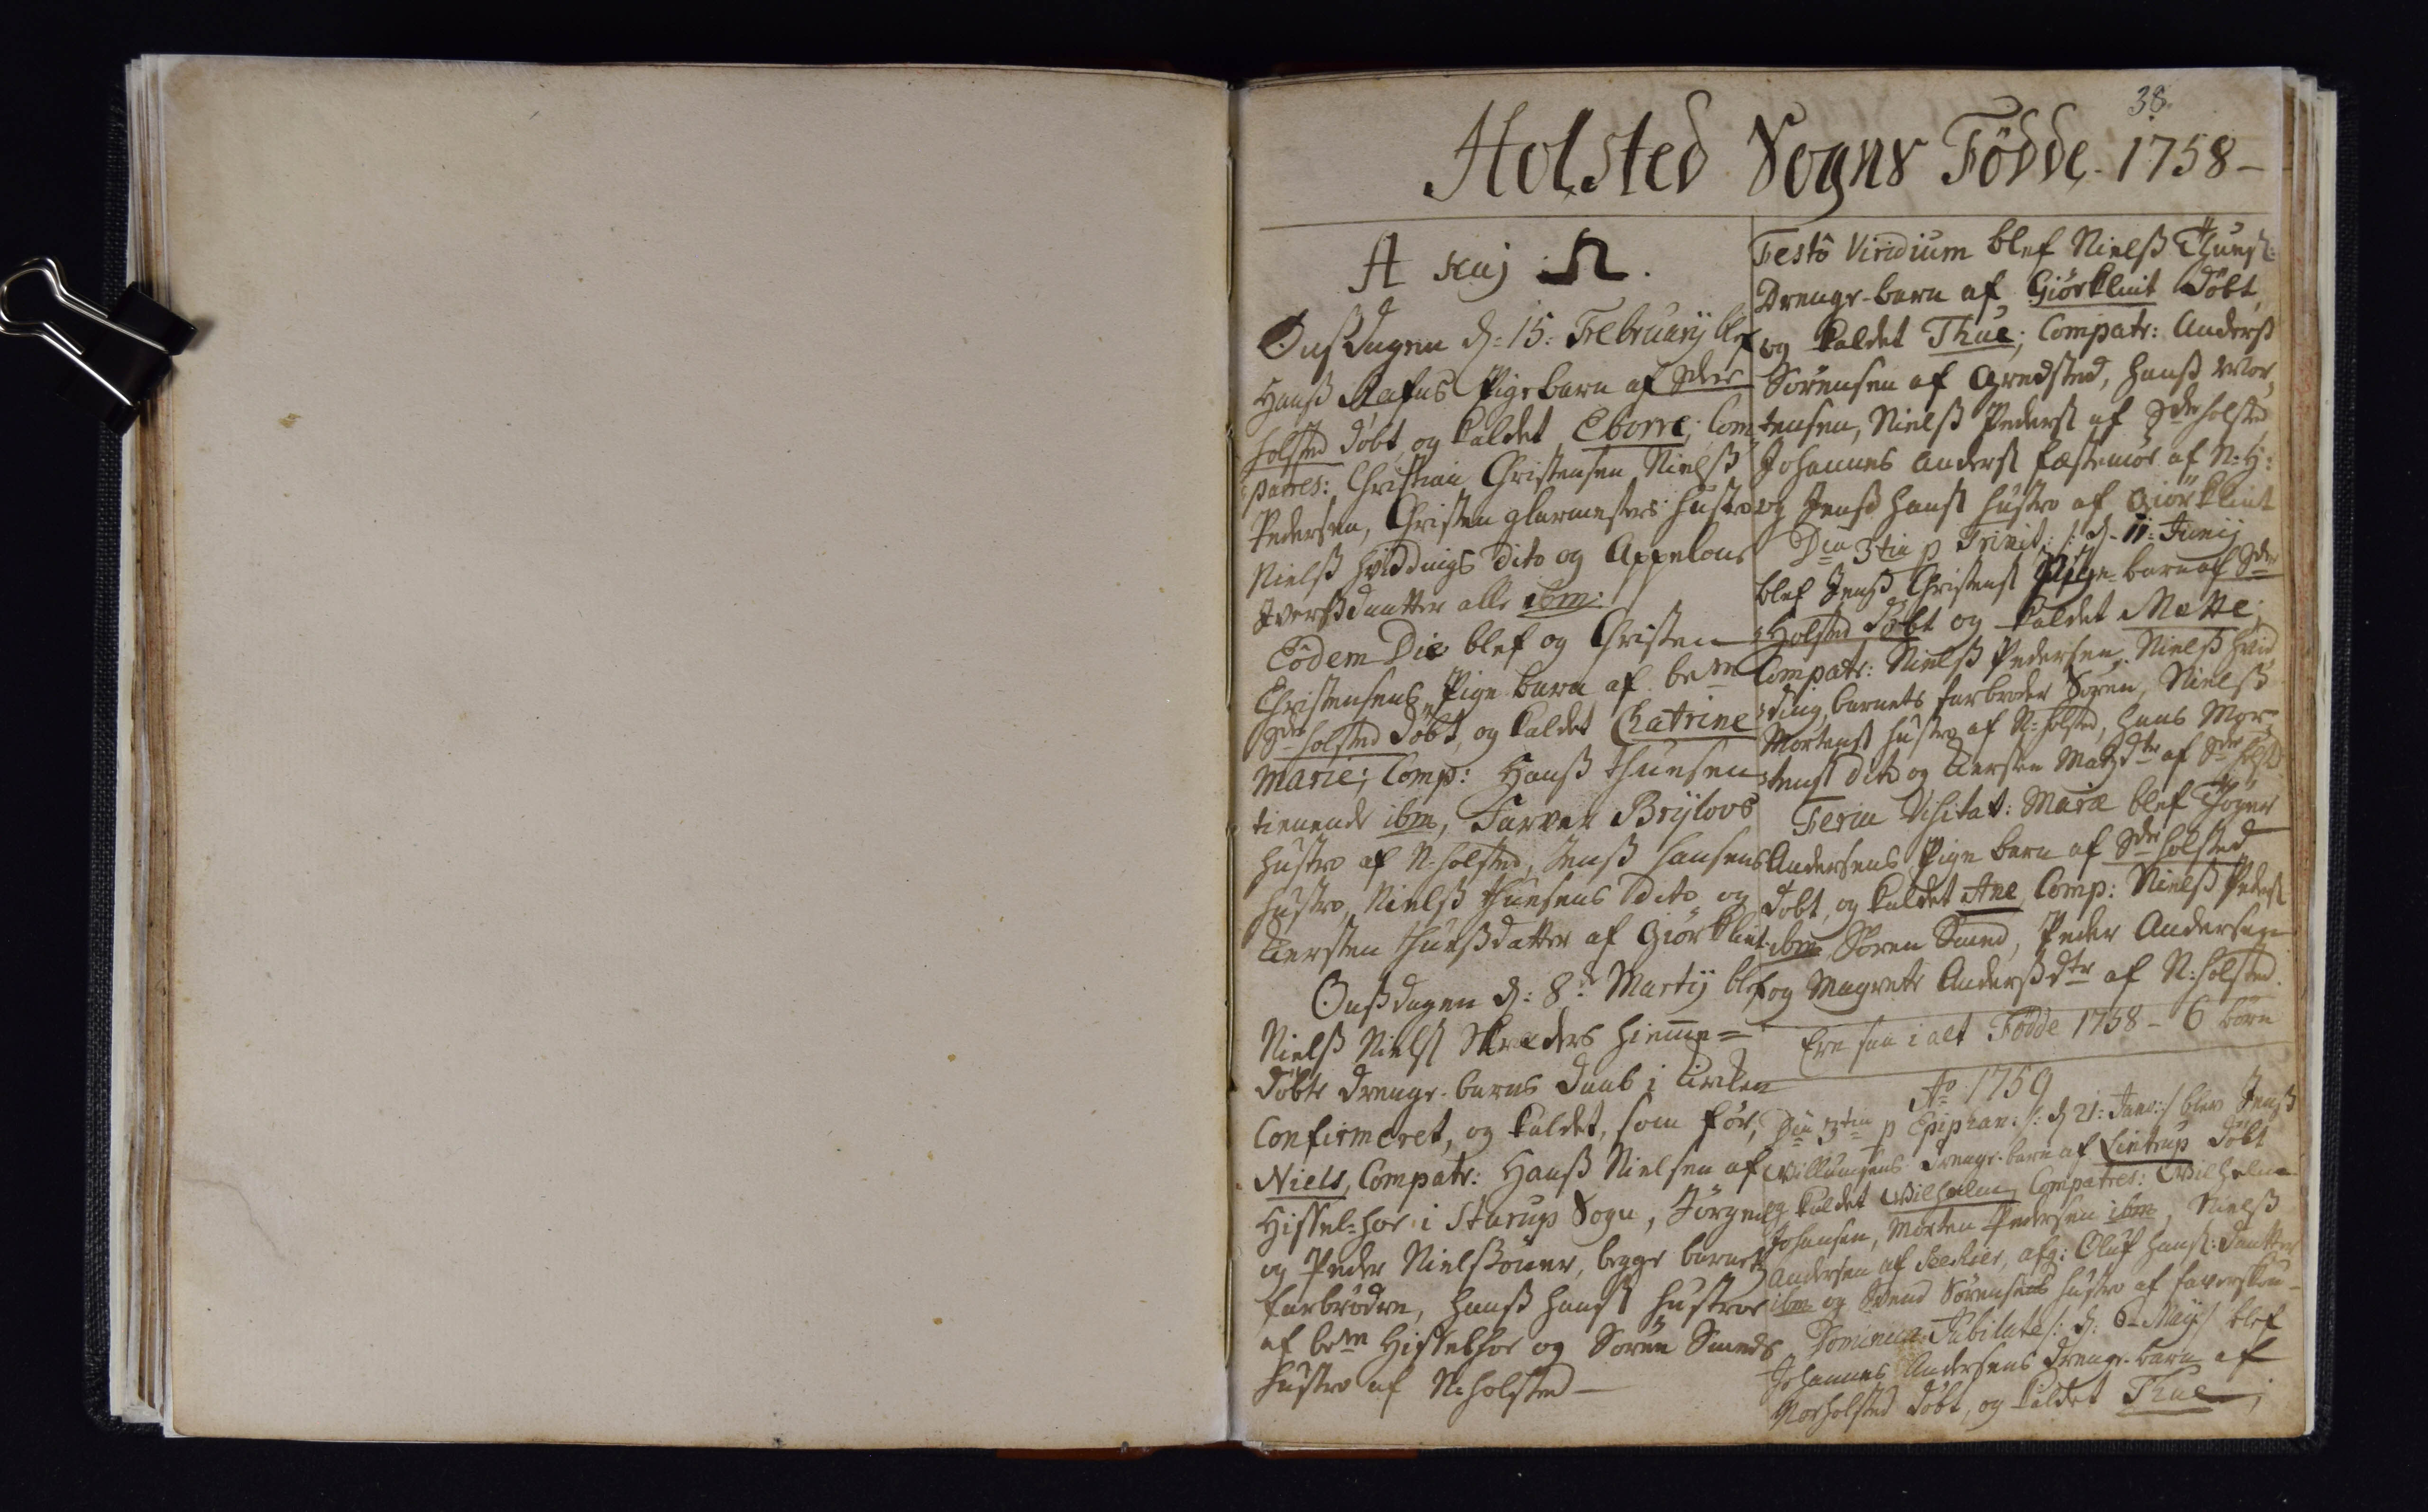Holsted Sogns Fødde. 1758 -
A Kaj Ω
Onssdagen d: 15: Februarij blef
Hans Rafns Pigebarn af Sdr
holsted døbt og kaldet Eborre; Com¬
parres: Christian Christensen Nielss
Pedersen, Christen glarmesters Hustro
Niels hviddings dito og Appelone
Iverfsdaatter alle ibm:
Eodem Die blef og Christen
Christensens Pige barn af bem
Sdr
holsted døbt og kaldet Chatrine
Marie; Comp: Hanss thuesens
tienende ibm: Farver Brylovs
Hustro af N: holsted, Jenss Hansens
Hustro Nielss Thuesens dito og
Kiersten Tuessdatter af Giørklint
Onsdagen d: 8de Martij blev
Nielss Nielss Skræders hiemme¬
døbte Drenge-barns daab i Kirken
Confirmeret, og kaldet, som før,
Niels Compatr: Hanss Nielsen af
Hissel=hoe i Starup Sogn; Jørgen
og Peder Nielsøner, begge barnets
farbrødre, Hanss Hanss Hustroe
af bem Hisselhoe og Søren Smeds
hustru af N: holsted -
38.
Festo Viridium blef Nielss Thues:
Drenge-barn af Giørklint døbt,
og kaldet Thue, Compatr: Anderss
Sørensen af Gredsted, Hans Mor¬
tensen, Niels Peders af Sdr holsted
Johannes Anders fæstemøe af N: H:
og Jenss Hans Hustro af Giørklint
Dca 3tia p Trinit: /: d. 11: Junij
blef Jenss Christens Pige-barn af Sdr
Holsted døbt og kaldet Mette;
Dompatr: Nielss Pedersen, Nielss Hvid¬
ding, barnets farbroder Soren, Niels
Mortens Hustro af N: holsted, Hans Mor¬
tens dito og Kirsten Madzdtr af Sdr holsted
Feria Visitat: Mariæ blef Thøger
Andersens Pige barn af Sdr holsted
døbt og kaldet Ane Comp: Niels Peders
ibm Søren Smed, Peder Andersen
og Magrete Andersdtr af N: holsted
Ere saa i alt Fødde 1758 - 6. børn
Ao 1759
Dca 3tia p Epiphan: /: d 21 Janv: :/ blev Jenss
Villumsens Drenge-barn af Lintrup døbt
og kaldet Wilhelm, Compatres. Wilhelm
Johansen, Morten Pedersen ibm; Nielss
Andersen af Seekier, afg: Oluf Hanss: Daatter
ibm og Svend Sørensens Hustro af faverskou
Dominica Jubilate /: d: 6 Maij blef
Johannes Andersens Drenge-barn af
Norholsted døbt, og kaldet Thue;
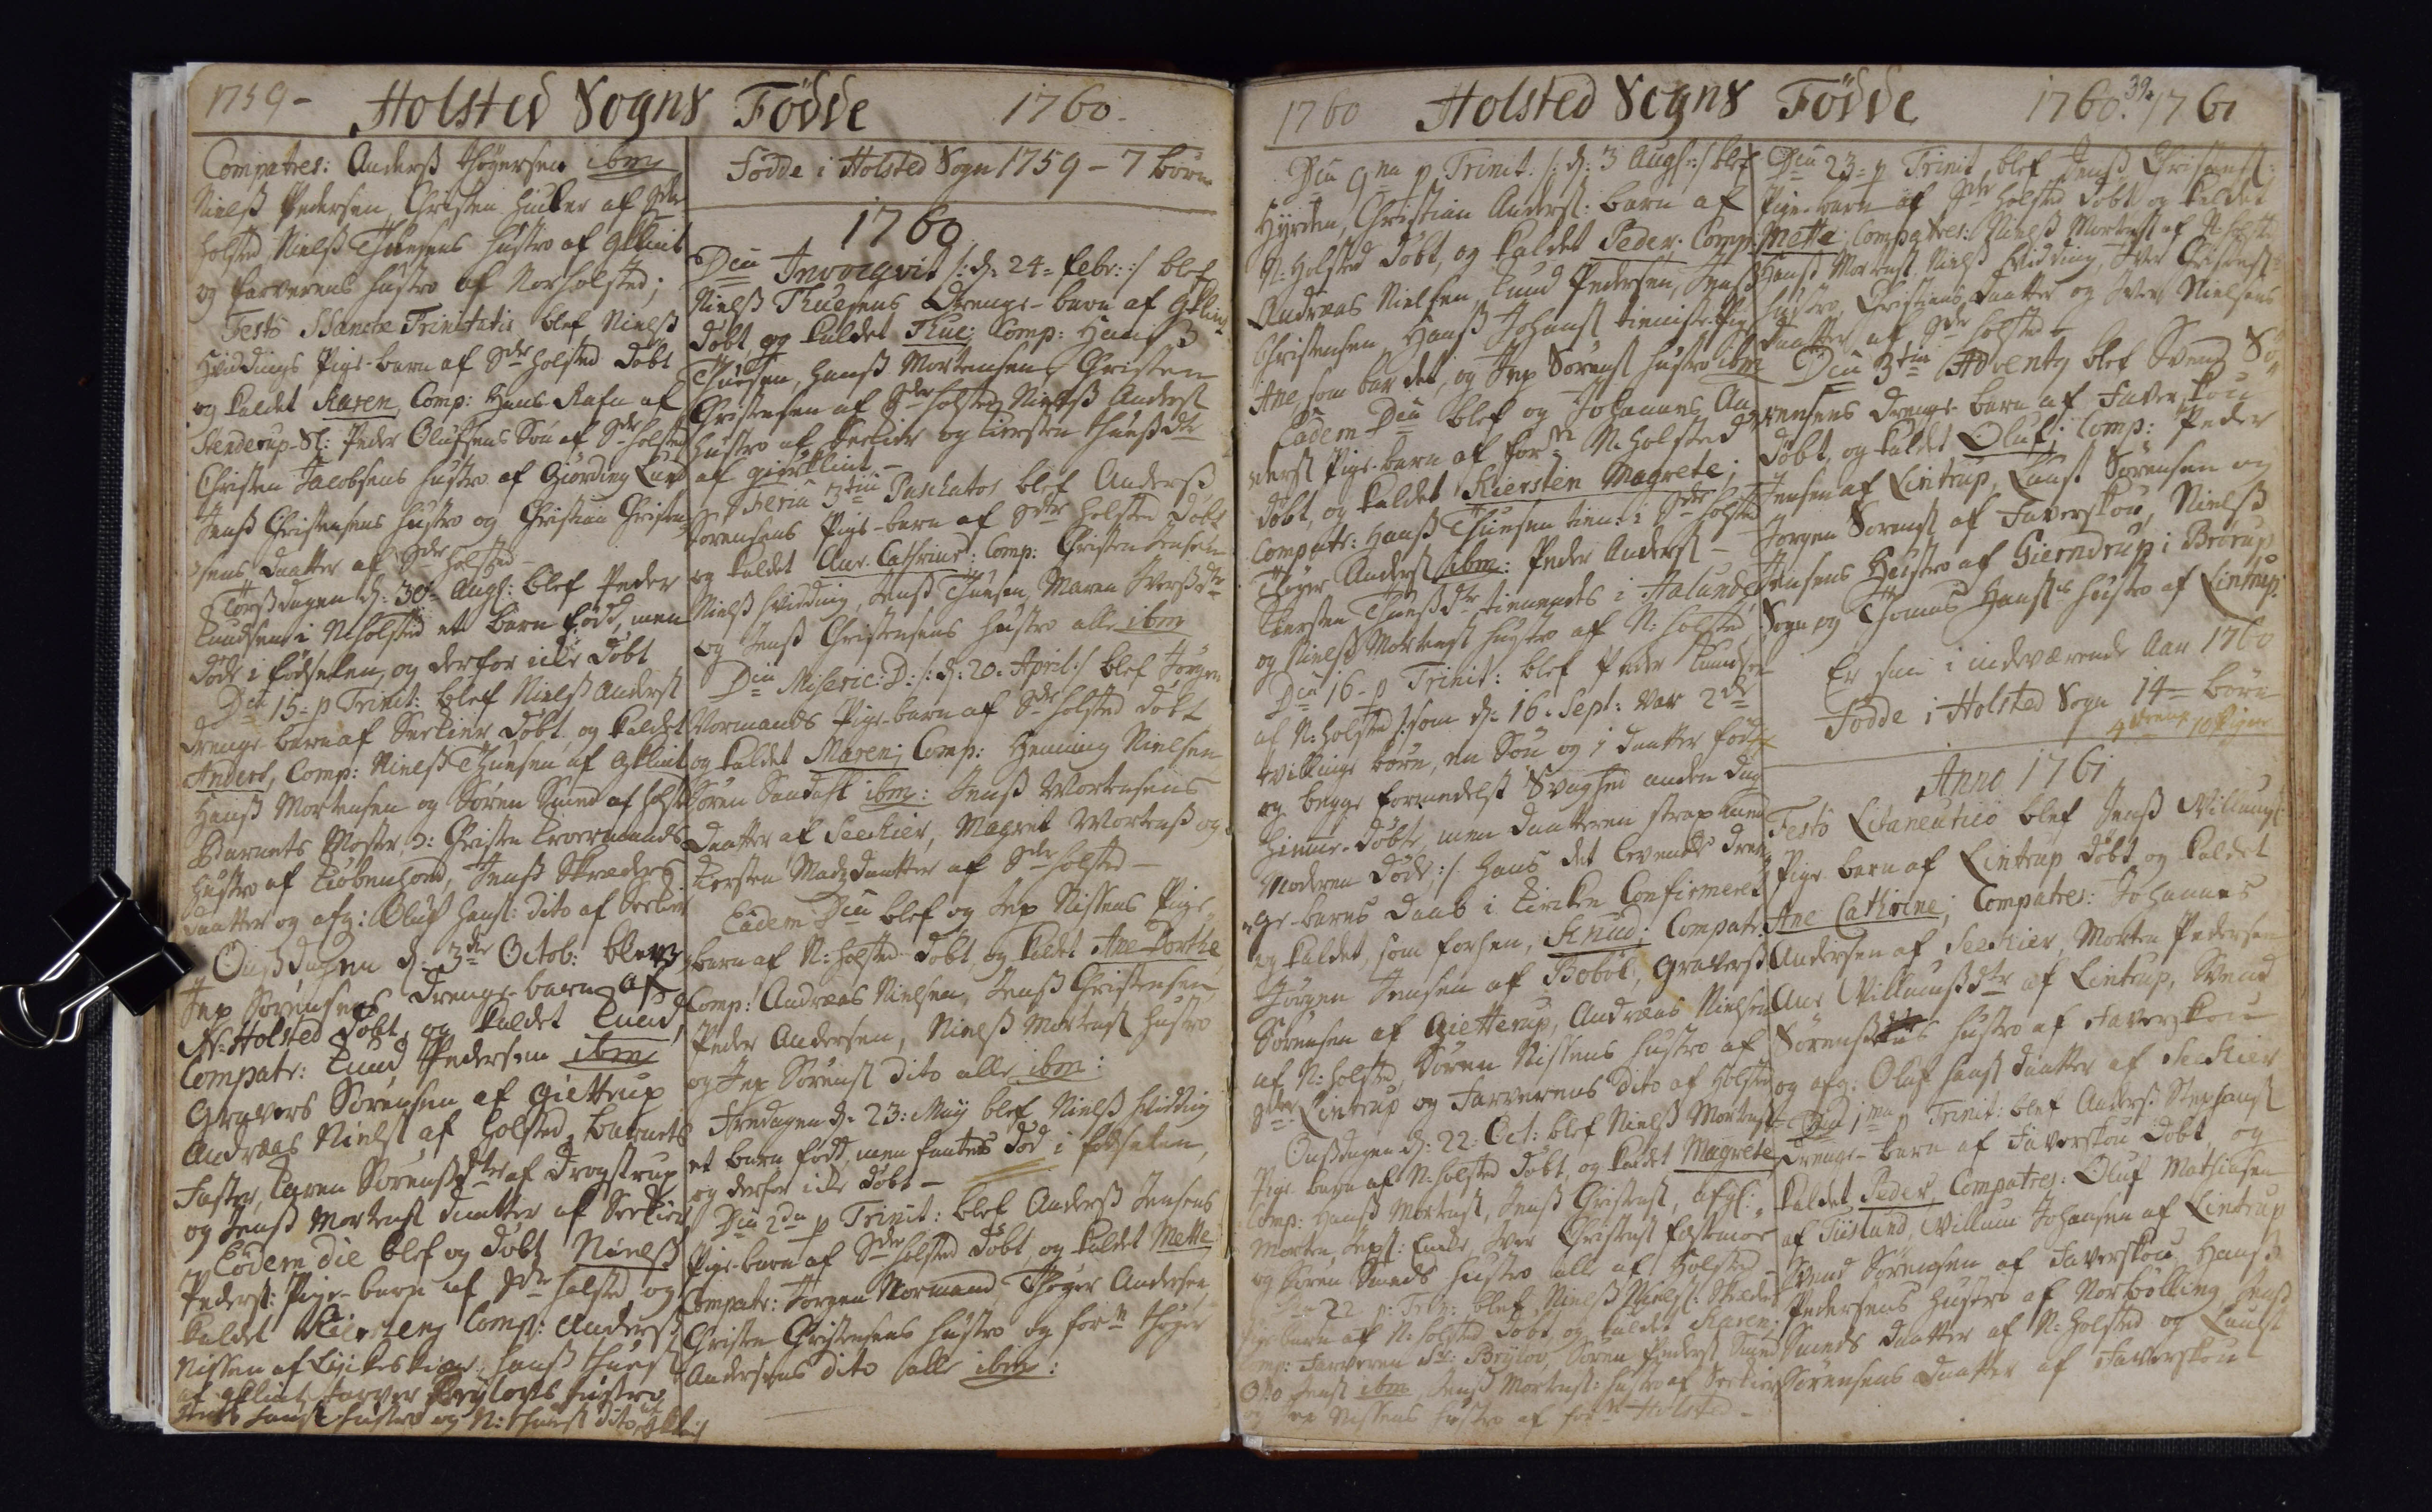1759 -
Holsted Sogns Fødde
Compatres: Anders Thøgersen ibm
Nielss Pedersen Christen Hiuler af Sdr
Holsted Niels Thusens Hustro af Gklint
og farverens Hustro af Nor Holsted
Festo SSancte Trinitatis blef Nielss
Hviddings Pige-barn af Sdr Holsted døbt
og kaldet Karen, Comp: Hans Nafn af
Stenderup - Sl: Peder Olufsens Søn af Sdr Holsted
Christen Jacobsens Hustro af Giørding Lund
Henss Christensens Hustro og Christian Christen¬
sens Daatter af Sdr Holsted -
Torssdagen d: 30: Aug: blef Peder
Knudsen i N Holsted et barn fødd, men
døde i Fødselen og derfor icke døbt
Dca 15: p Trinit: blef Nielss Anders
Drenge-barn af Serkier døbt og kaldet
Anders, Comp: Niels Thuesen af Gklint
Hanss Mortensen og Søren Smed af Holsted
Barnets Moster, ɔ: Christen Kroermands
Hustro af Kiøbenhoed, Jenss Skræders
Daatter og afg: Oluf Hans: dito af Serkier
Onssdagen d: 3die Octob: blev
Jep Sørensens Drenge-barn af
N=Holsted døbt og kaldet Kund
Compatr: Knud Pedersen ibm
Grevers Sørensen af Giettrup
Andreas Niels af Holsted barnet
Faster, Karen Sørensdtr af Drogstrup
og Jenss Mortens Daatter af Serkier
Eodem Die blef og døbt Nielss
Peders: Pige-barn af Sdr Holsted og
kaldet Kiersten Dom: Anderss
Nissen af Lyckeskiær Hanss Thues
af Gklint, farver Brylovs Hustro
af
Jens Hans Hustro og N: Hans dito Gklint
1760
Fødde i Holsted Sogn. 1759 - 7 Børn
1760
Dca Invocavit /: d: 24= Febr: :/ blef
Nielss Thuesens Dreng-barn af Gklint
døbt og kaldet Thue Comp: Hanss
Thuesen, Hans Mortensen, Christen
Christensen af Sdr Holsted Nielss Anders
Hustro af Serlier og Kirsten Thuessdtr
af Giørklint. 
Feria 3tia Paschatos blef Anderss
Sørensens Pige-barn af Sdr Holsted døbt,
og kaldet Ane Cathrine: Comp: Christen Jensen
Niels Hvidding, Jens Thuesen Maren Iversdtr
og Jenss Christensens Hustro alle ibm
Dca Miseric: D: /: d: 20: April :/ bleff Thøger
Normands Pige-barn af Sdr Holsted døbt
og kaldet Maren; Comp: Henning Nielsen
Søren Sandahl ibm: Jens Mortensens
Daatter af Seekier Magret Mortenss og
Kiersten Madzdaatter af Sdr Holsted -
Eodem Dca blef og Jep Nissens Pige
barn af N: Holsted døbt, og kaldet Ane Dorthe
Comp: Andræas Nielsen, Jenss Christensen
Peder Anderssen, Niels Mortensen Hustro
og Jep Sørens dito alle ibm
Fredagen d: 23: Maij bleff Niels Hvidding
et Barn fødd men fanters død i Fødselen
og derfor icke døbt -
Dca 2da p Trinit: blef Anderss Jensens
Pige-barn af Sdr Holsted døbt, og kaldet Mette
Compatr: Jørgen Normand, Thøger Andersen
Christen Christensens Hustro og forn Thøger
Andersens Dito alle ibm:
1760 Holsted Sogns Fødde
Dca 9na p Trinit: /: d: 3 Aug##: :/ blef
Hyrden, Christian Anders: barn af
N: Holsted døbt, og kaldet Peder. Comp:
Andræas Nielsen, Knud Pedersen, Jenss
Christensen Hanss Johans tieniste-Pige
Ane, som bar det, og Jep Sørens Hustro ibm
Eodem Dca blef og Johannes An
ders Pige-barn af forn N. Holsted
døbt, og kaldet Kiersten Magrete;
Comptr: Hanss Thuesen tien: i Sdr Holsted
Thøger Anders ibm; Peder Anders
Kirsten Thuessdtr tienendes i Aalunde
og Nielss Mortens Hustro af N: Holsted
Dca 16 - p Trinit: blef Peder Knuds
af N: Holsted /.  som d: 16. Sept: var 2de
tvillinge børn, en Søn og 1 Daatter fødd
og begge formedelst Svaghed anden dag
Hiemme-døbte, men daatteren strax ##
Moderen døde: Hans det levende Dren¬
ge-barns Daab i Kirken Confirmeret
og kaldet, som forhen Knud Compatr:
Jørgen Jensøn af Bobøl; Graver
Sørensen af Gietterup, Andræas Nielsen
af N: Holsted Søren Nissens Hustro af
Sdr Lintrup og Farverens Dito af Holsted
Onssdagen d: 22 Oct: blef Niels Mortens
Pige barn af N: Holsted døbt, og kaldet Margrete
Comp: Hanss Mortens, Jenss Christens, afgl:
Morten Jeps: Enke Iver Christens Fæstemøe
og Søren Smeds Hustro alle af Holsted
Dca 22 p: Trin: blef Nielss Niels: Skræders
Pige barn af N: Holsted døbt, og kaldet Karen;
Comp: Farveren Sr: Brylov, Søren Peders Svend
Otto Jens ibm Jenss Mortens: Hustro af Serkier
og Jep Nissens Hustro af forn Holsted
39.
1760.1761
Dca 23= p Trinit, blef Jenss Christens:
Pige-barn af Sdr Holsted døbt og kaldet
Mette Compatres: Nielss Mortens af N: Holsted
Hans Mortens, Niels Hvidding, Iver Christens:
Hustro, Christians Daatter og Iver Nielsens
Daatter af Sdr Holsted
Dca 3tia Adventy blef Svens Sø¬
rensens Drenge-barn af Faverskou
døbt, og kaldet Oluf; Comp: Peder
Jensen af Lintrup, Laus Sørensøn og
Jorgen Sorens af Faverskou Nielss
Jensens Hustro af Gierndrup i Brørup
Sogn og Thomas Hanss Hustro af Lintrup
Er som i indeværende Aar 1760
Fødde i Holsted Sogn 14= Børn
1 Drenge 10 Piger
Anno 1761
Festo Litaneutico blef Jens Villums:
Pige Barn af Lintrup døbt og kaldet
Ane Cathrine; Compatre: Johannes
Andersen af Seekier, Morten Pedersen
Ane Villumssdtr af Lintrup, Svend
Sørenssens Hustro af Faverskou
og afg: Oluf Hans daatter af Seekier
Dca 1ma p Trinit: blef Anders Stephans
Dreng= barn af Faverskou døbt, og
kaldet Peder, Compatres: Oluf Mathiesen
af Tiislund, Villum Johansen af Lintrup
Svend Sørrensen af Faverskou, Hanss
Pedersens Hustro af Nor,bølling, Jens
Smeds Daatter af N: Holsted og Laus
Sørensens Daatter af Faverskou